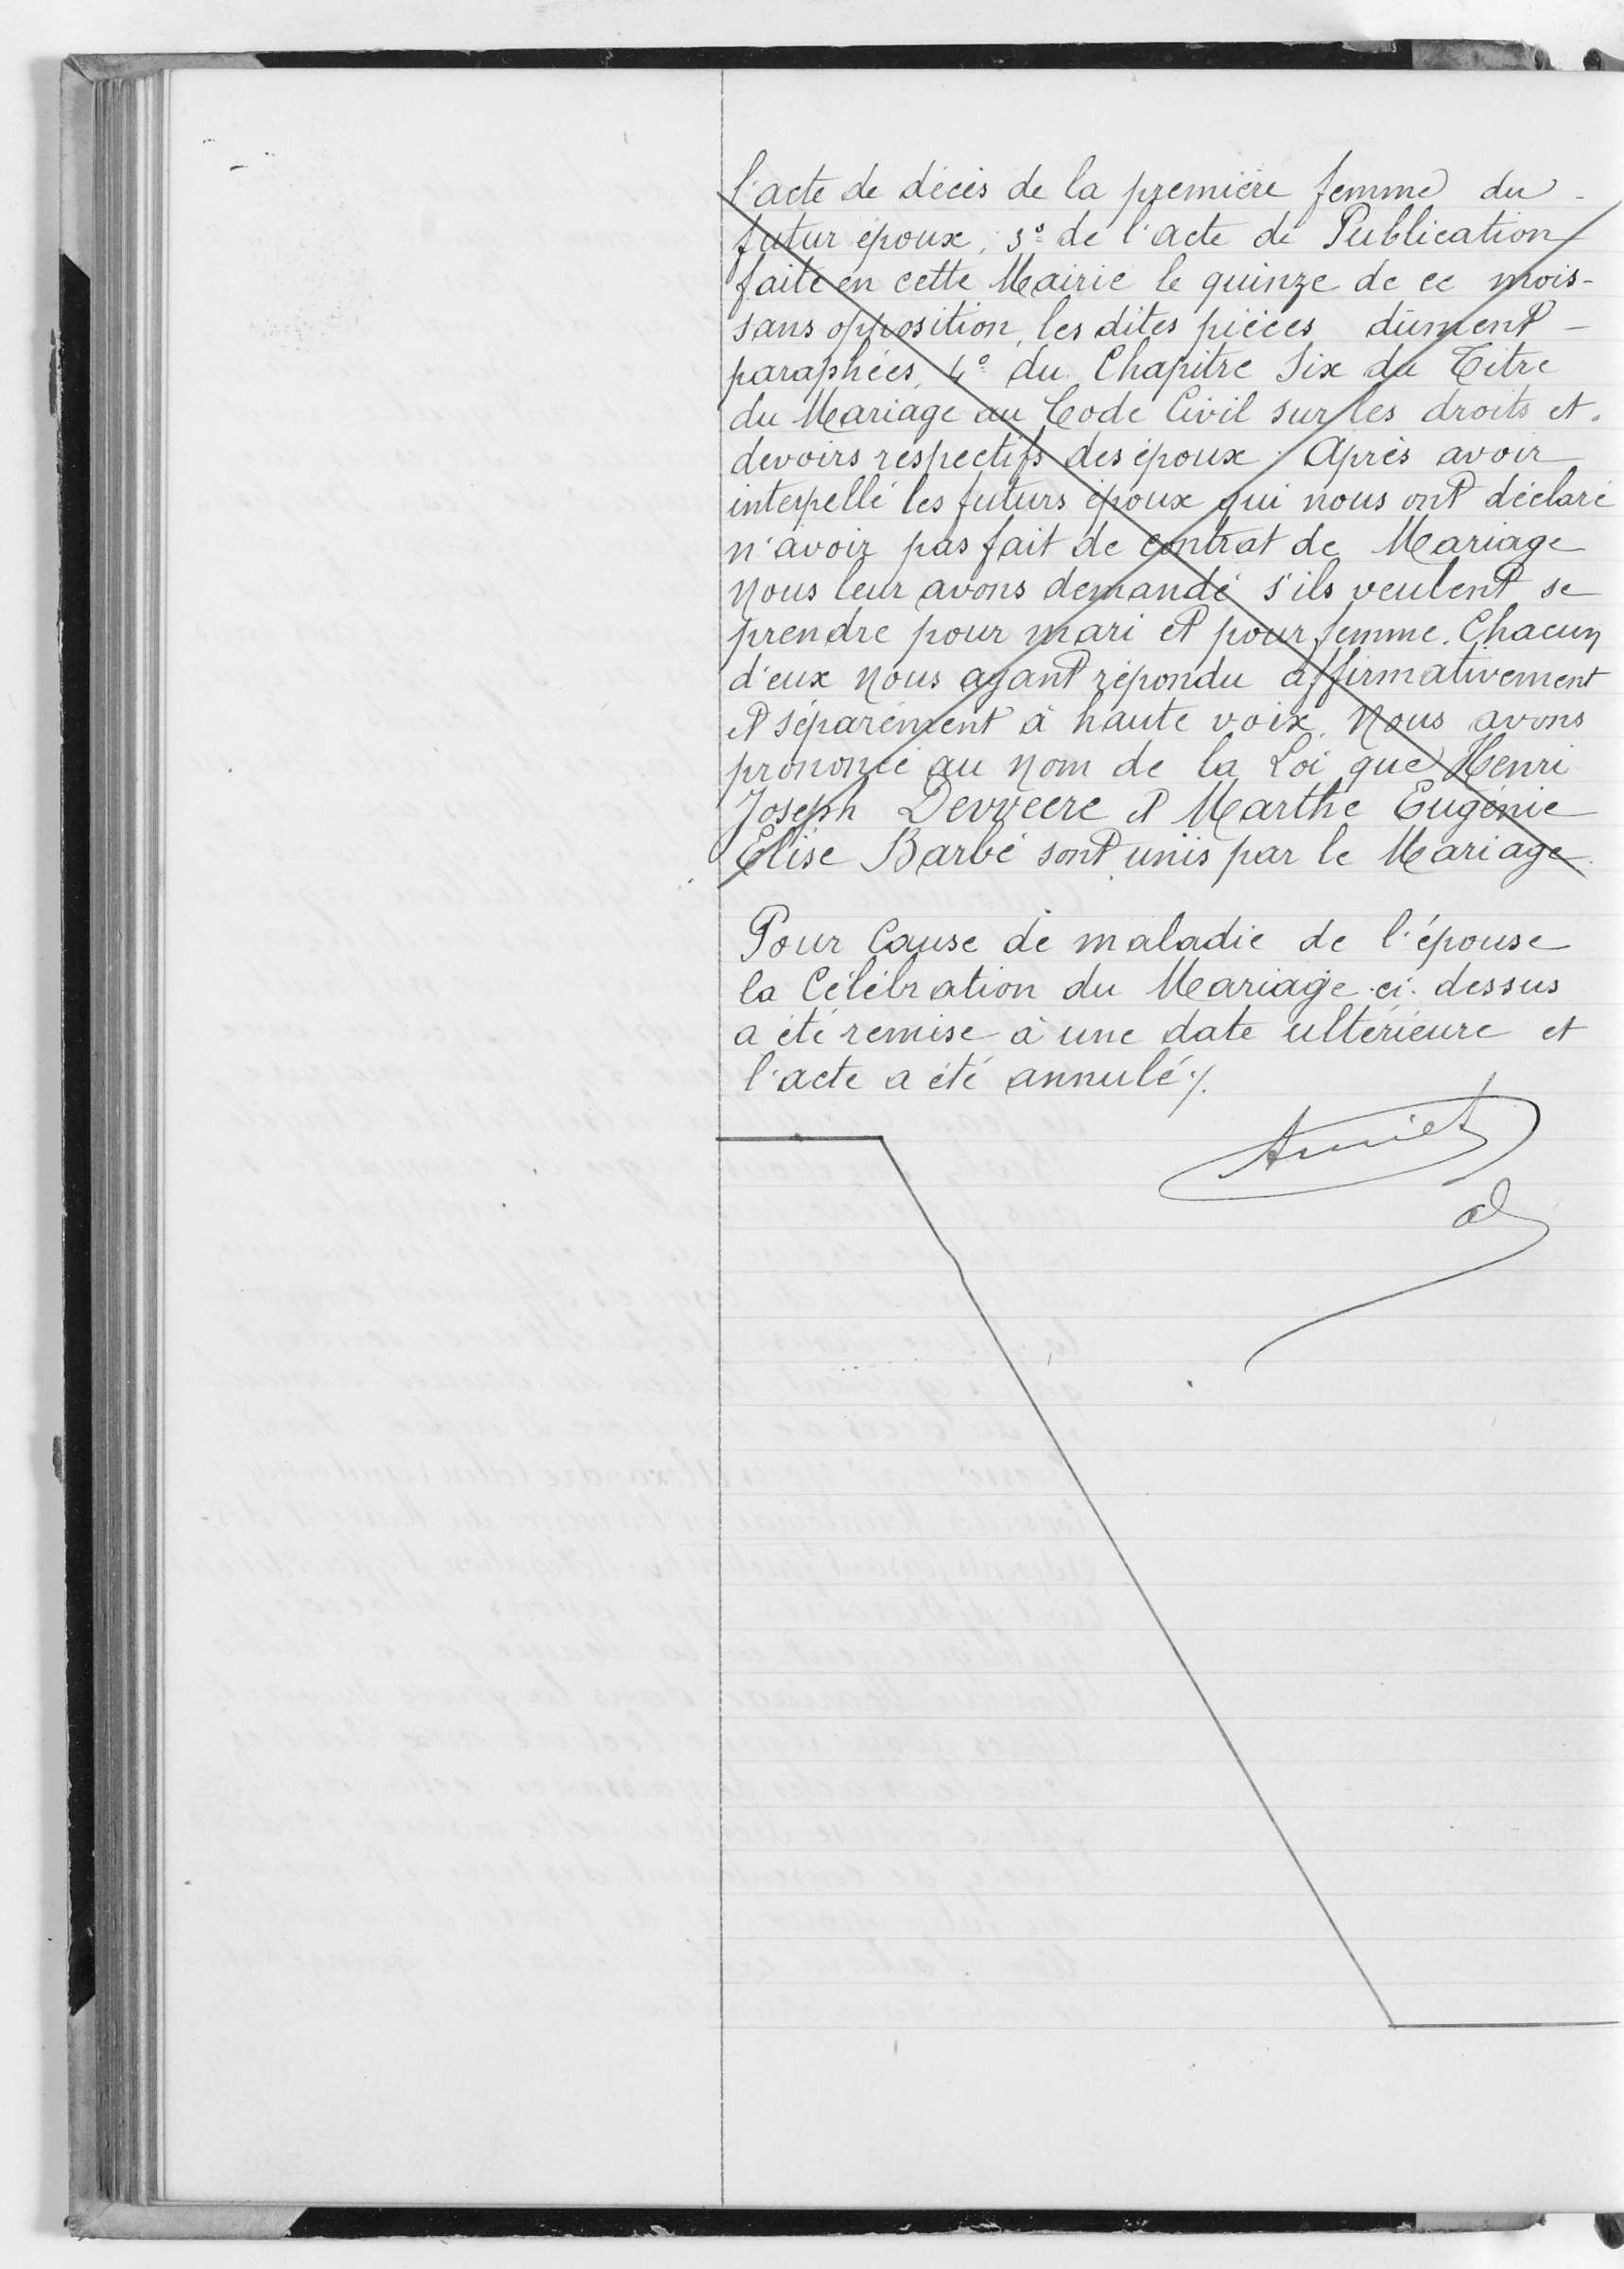l'acte de dècès de la première femme du
futur époux, 3° de l'acte de Publication
faite en cette Mairie le quinze de ce mois
sans opposition, les dites pièces dûment
paraphées, 4° du Chapitre Six du Titre
du Mariage au Code Civil sur les droits et
devoirs respectifs des époux. Après avoir
interpellé les futurs époux qui nous ont déclaré
n'avoir pas fait de contrat de Mariage
nous leur avons demandé s'ils veulent se
prendre pour mari et pour femme. Chacun
d'eux nous ayant répondu affirmativement
et séparément à haute voix. Nous avons
prononcé au nom de la Loi que Henri
Joseph Devveere et Marthe Eugénie
Elise Barbe sont unis par le Mariage
Pour cause de maladie de l'épouse
la Célébration du Mariage ci-dessus
a été remise à une date ultérieure et
l'acte a été annulé ./.
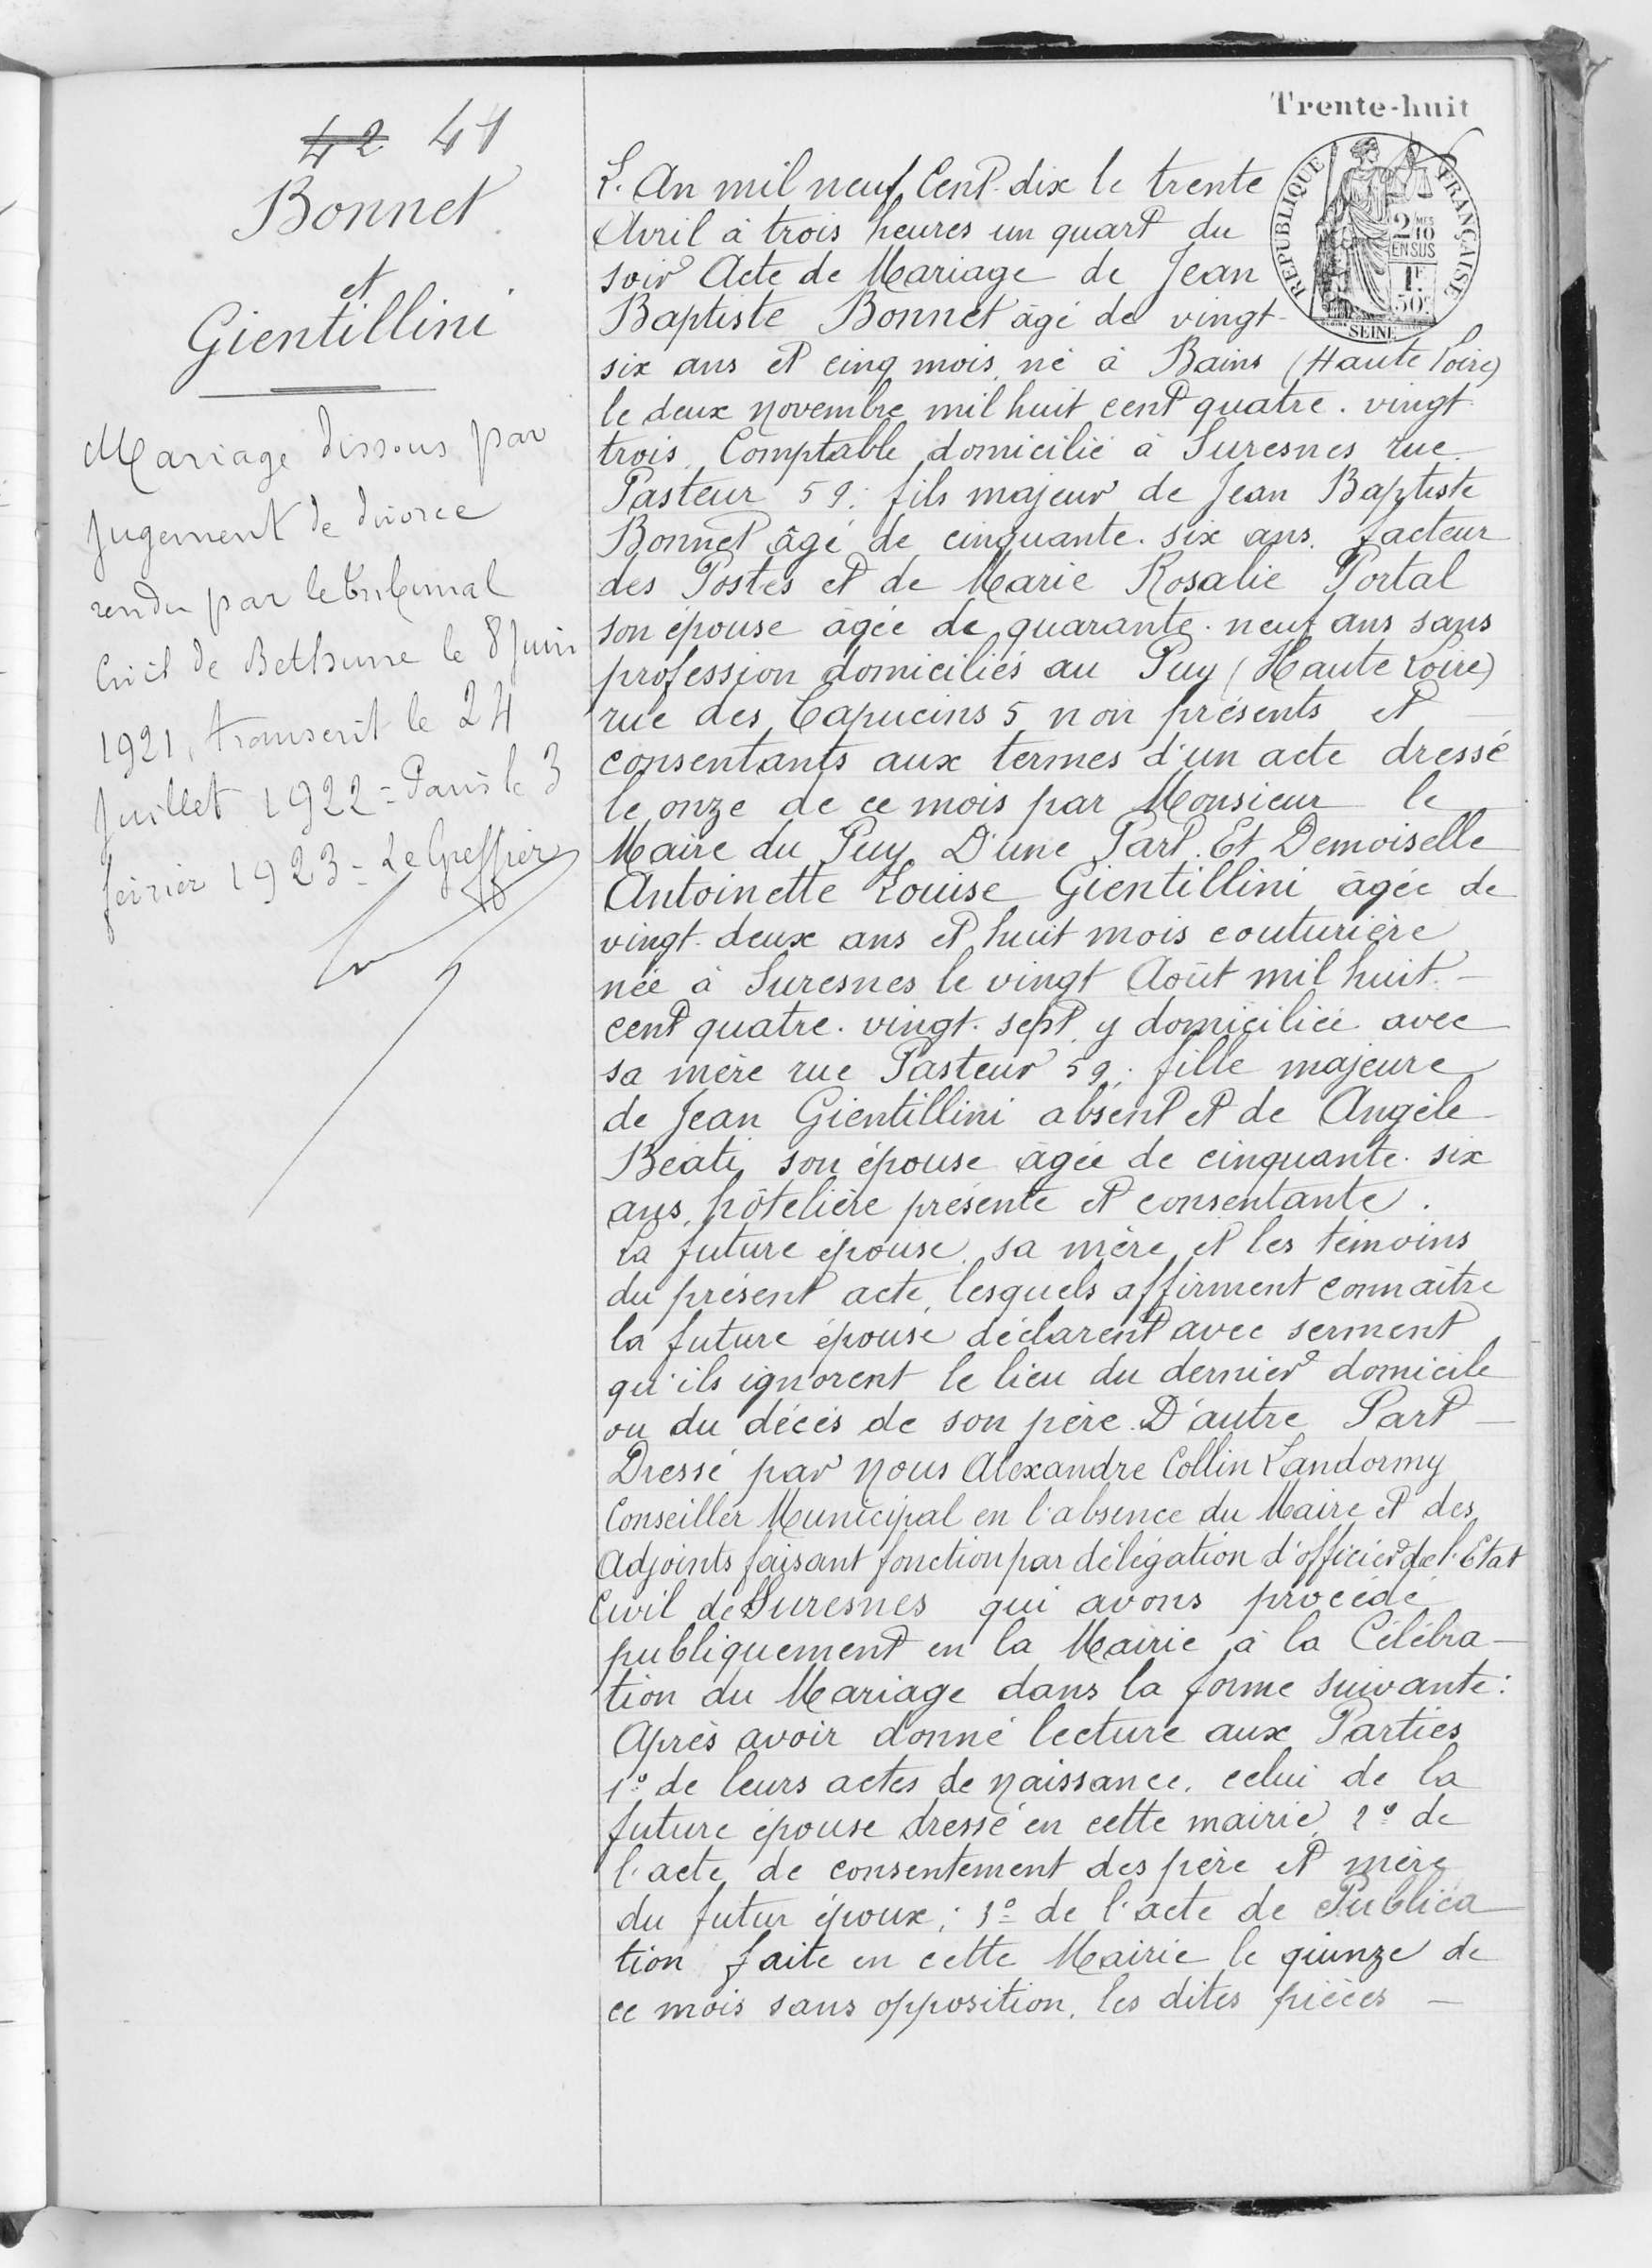41
Bonnet
et Gientillini
L'an mil neuf cent dix le trente
Avril à trois heures un quart du
soir Acte de mariage de Jean
Baptiste Bonnet âgé de vingt
six ans et cinq mois né à Bains (Haute Loire)
le deux novembre mil huit cent quatre vingt
trois comptable domicilié à Suresnes rue
pasteur 59, fils majeur de Jean Baptiste
Bonnet âgé de cinquante six ans facteur
des Postes et Marie Rosalie Portal
son épouse âgée de quarante-neuf ans sans
profession domiciliés au Puy (Hautre-Loire)
rue des Capucins 5 non présents et
consentants aux termes d'un acte dressé
le onze de ce mois par Monsieur le
Maire du Puy. D'une Part Et Demoiselle
Antoinette Louise, Gientillini âgée de
vingt-deux ans et huit mois couturière
née à Suresnes le vingt août mil huit
cent quatre vingt-sept y domiciliée avec
sa mère rue Pasteur 59; fille majeure
de Jean Gientillini absent et de Angèle
Beati son épouse âgée de cinquante-six
ans, hôtelière présente et consentante.
La future épouse sa mère et les témoins
du présent acte, lesquels affirment connaître
la future épouse déclarent avec serment
qu'ils ignorent le lieu du dernier domicile
ou du décès de son père. D'autre Part-
Dressé par nous Alexandre Collin Landormy
Conseiller Municipal en l'absence du Maire et des
adjoints faisant fonction par délégation d'officier de l'Etat
Civil de Duresnes qui avons procédé
publiquement en la Mairie à la Célébra-
tion du Mariage dans la forme suivante:
Après avoir donné lecture aux Parties
1° de leurs actes de naissance, celui de la
future épouse dressé en cette mairie 2° de
l'acte de consentement des père et mère
du futur époux; 3°: de l'acte de Publica
tion faite en cette Mairie le quinze de
ce mois sans opposition, les dites pièces